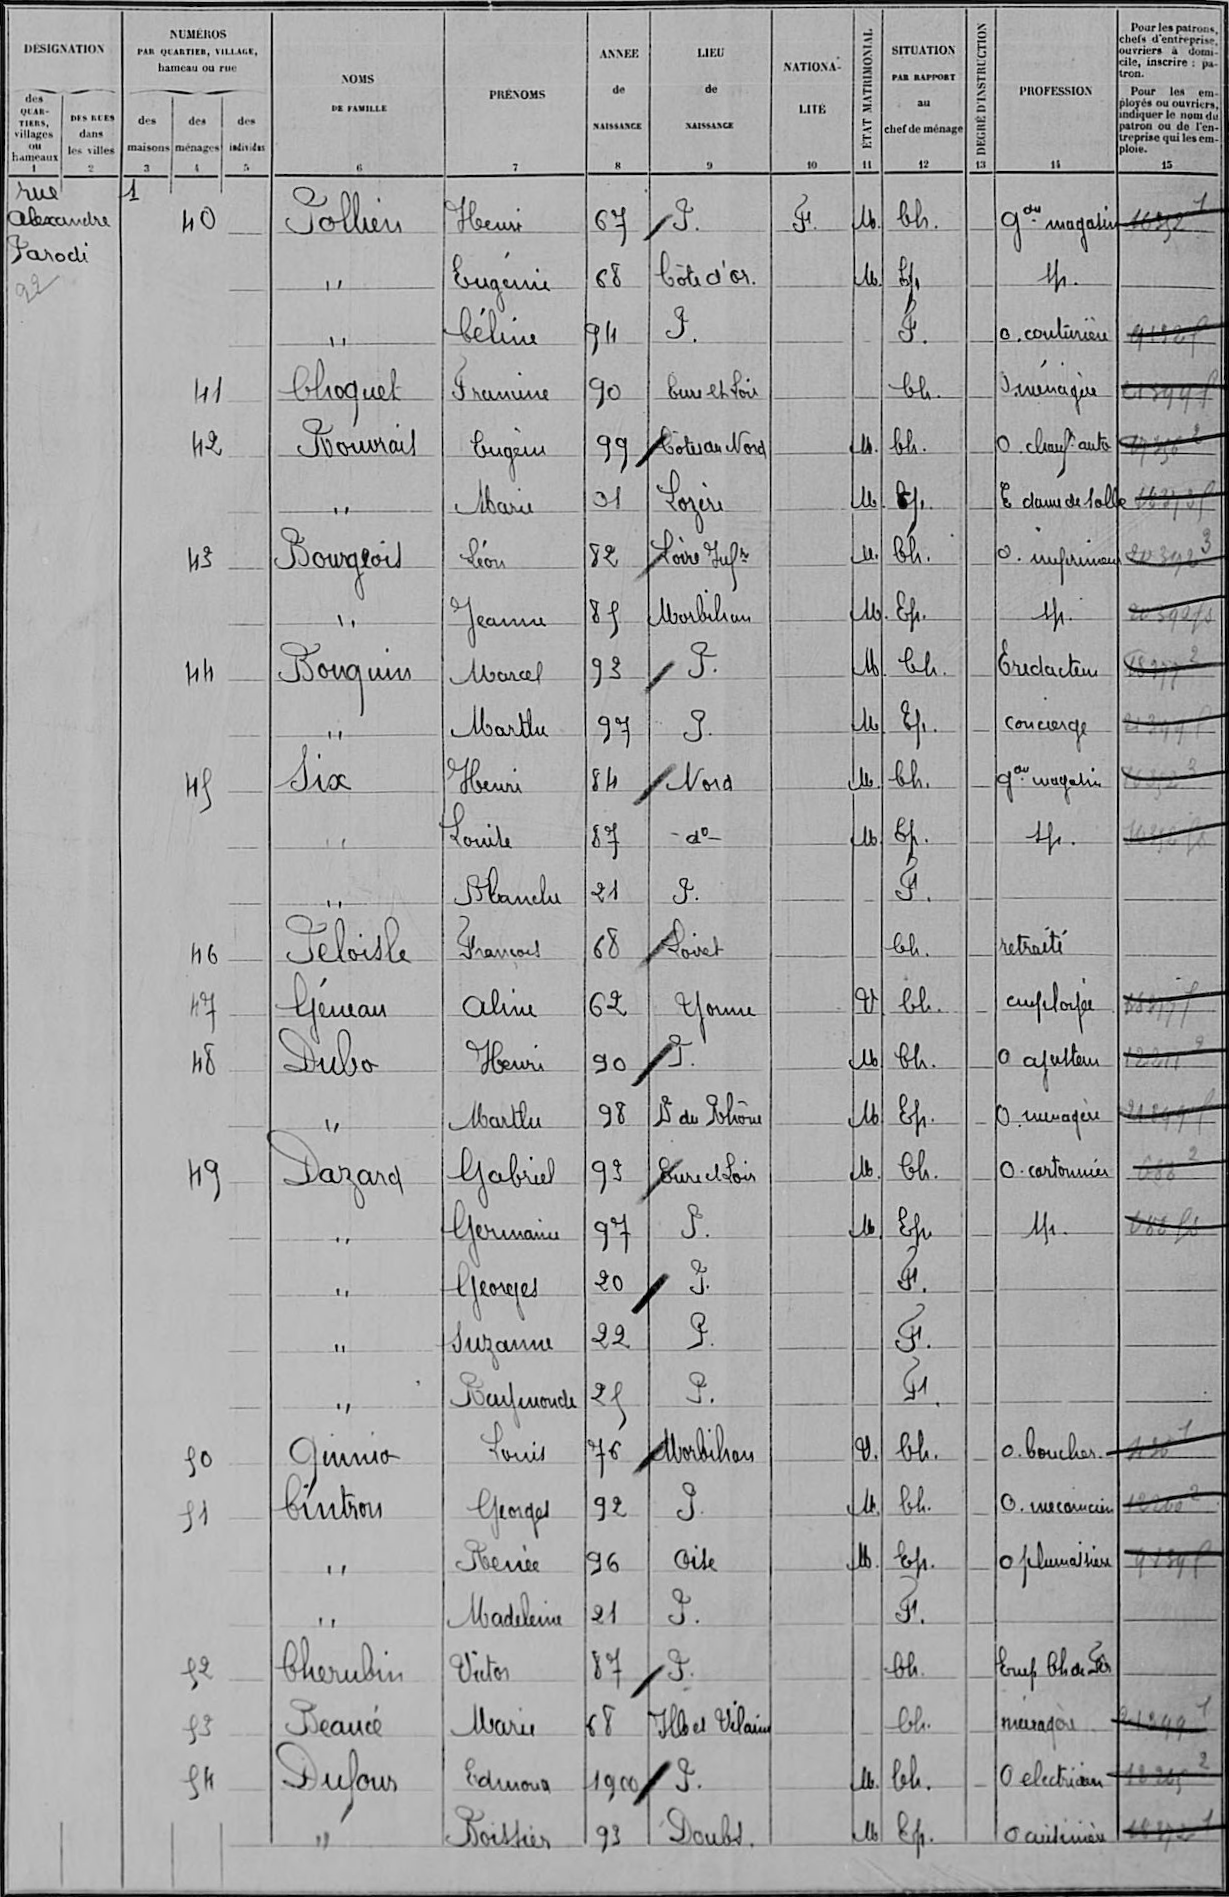Pollien/Henri/67/P /F /M /Ch /¤/Gon magasin/16352
"/Eugénie/68/Côte d'Or/¤/M /Ep/¤/sp /¤
¤/Céline/94/P /¤/¤/F /¤/o couturière/9132 f
Choquet/Francine/90/Eure et Loir/¤/¤/Ch /¤/o ménagère/21399 f
Rouvrais/Eugène/99/Côtes du Nord/¤/M /Ch /¤/O chauf auto/17356
¤/Marie/01/Lozère/¤/M /Ep /¤/E dame d salle/18373
Bourgeois/Léon/82/Loire Infre/¤/M/Ch /¤/o imprimeur/20392
¤/Jeanne/85/Morbihan/¤/M/Ep /¤/sp /20399 fs
Bouquin/Marcel/93/P /¤/M/Ch /¤/E redacteur/18377
¤/Marthe/97/P /¤/M/Ep /¤/concierge/21399 f
Six/Henri/84/Nord/¤/M /Ch /¤/gon magasin/16352
¤/Louise/87/d°/¤/M/Ep /¤/sp /16352
¤/Blanche/21/P /¤/¤/F /¤/¤/¤
Peloisle/François/68/Loiret/¤/¤/Ch /¤/retraité/¤
Géneau/Aline/62/Yonne/¤/V/Ch /¤/employée/18377
Dubo/Henri/90/P /¤/M /Ch /¤/o ajusteur/12277
¤/Marthe/98/B du Rhône/¤/M/Ep /¤/O menagere/21399 f
Dazard/Gabriel/93/Eure et Loir/¤/M /Ch /¤/O cartonnier/688
¤/Germaine/97/P /¤/M/Ep/¤/Sp /688 fs
"/Georges/20/P /¤/¤/F /¤/¤/¤
¤/Suzanne/2/P /¤/¤/F /¤/¤/¤
¤/Raymonde/25/P /¤/¤/F /¤/¤/¤
Ginnio/Louis/76/Morbihan/¤/V /Ch /¤/o boucher/436
Cintron/Georges/92/P /¤/M /Ch /¤/O mecanicien/12260
¤/Renée/96/Oise/¤/M /Ep /¤/o plumassière/9834 f
¤/Madeleine/21/P /¤/¤/F /¤/¤/¤
Cherubin/Victor/87/P /¤/¤/Ch /¤/Emp Ch de Fer/¤
Beaucé/Marie/68/Ille et Vilaine/¤/¤/Ch /¤/méangère/21399
Dufour/Edmond/1900/P /¤/M/Ch /¤/O elecricien/18205
¤/Boissier/93/Doubs/¤/M/Ep /¤/o cuisinière/18372
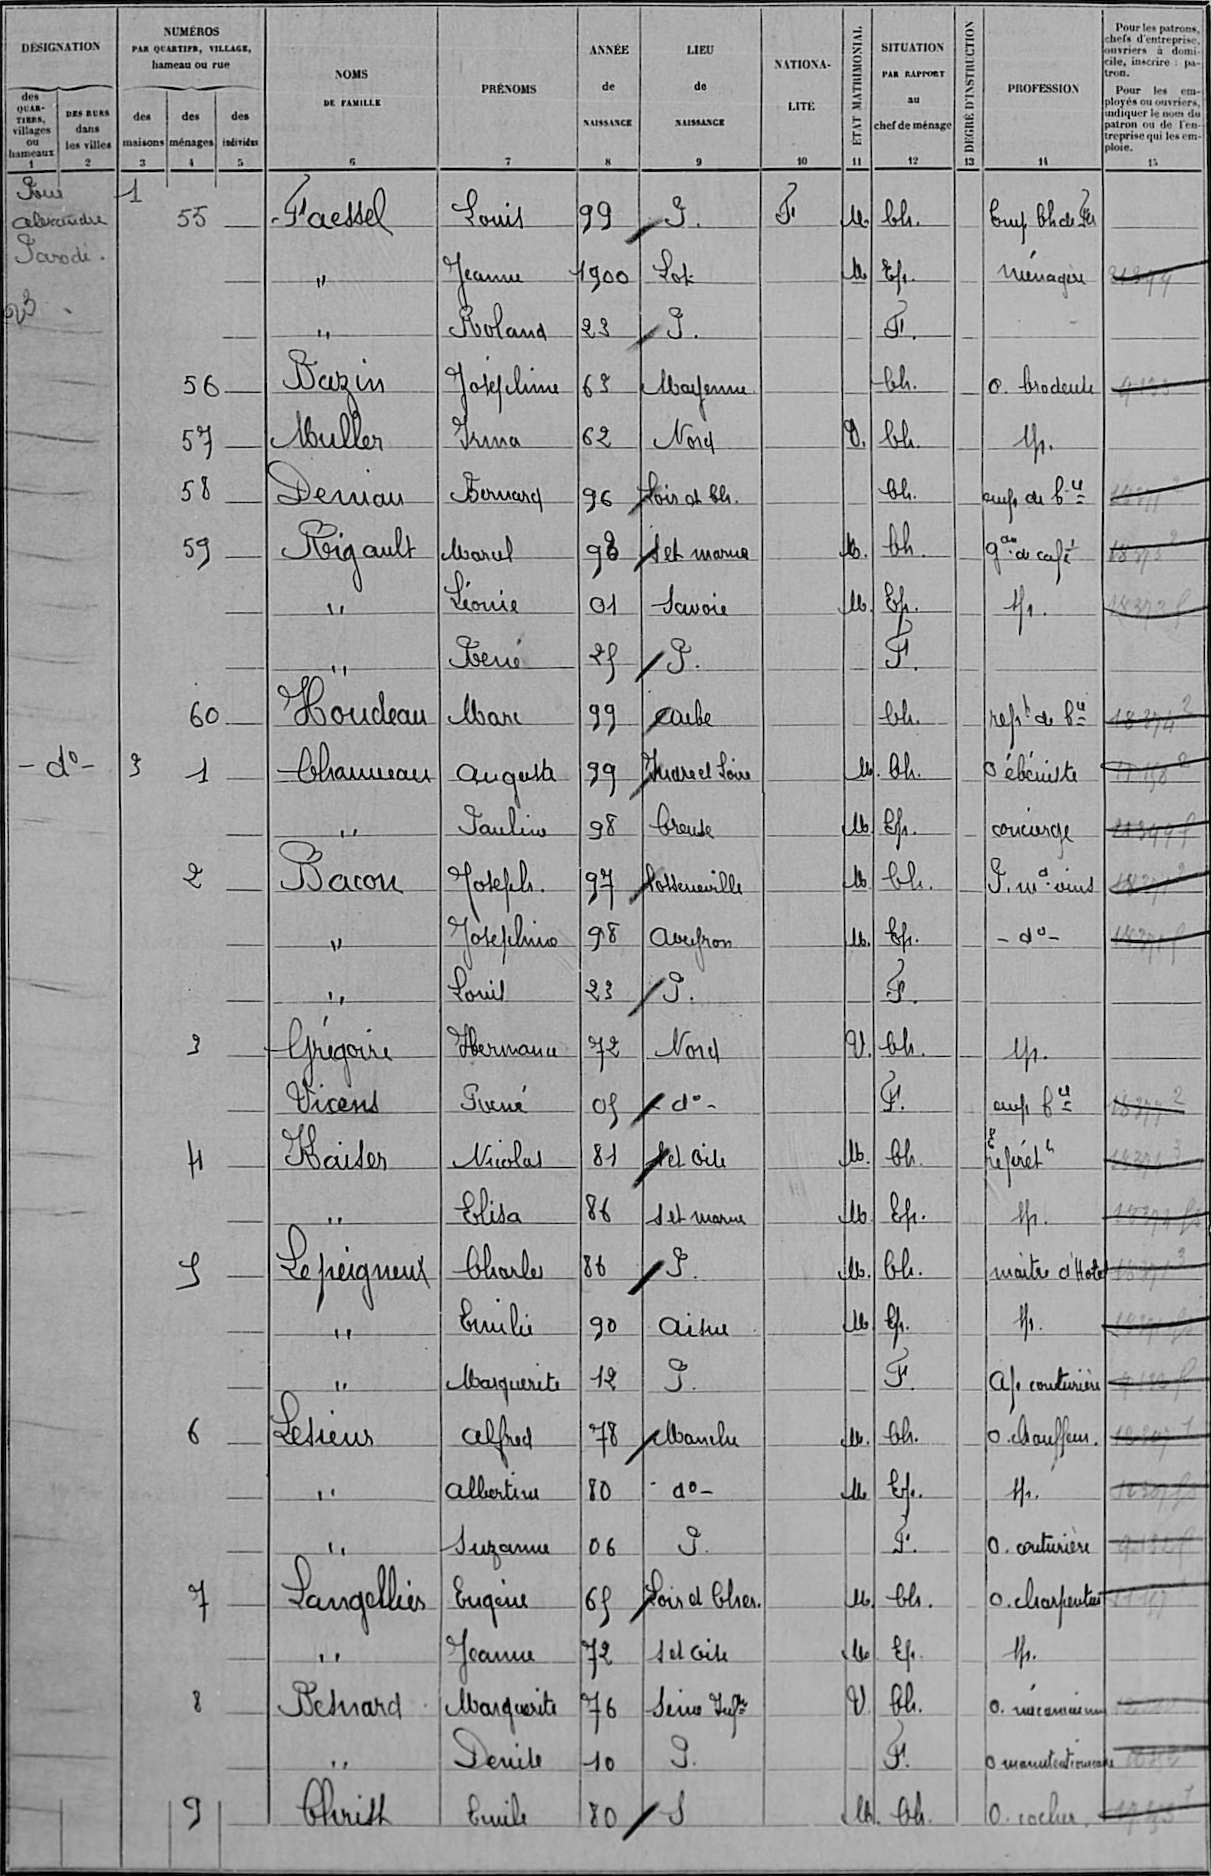Faessel/Louis/99/P /F/M /Ch /¤/Emp Ch de fer/¤
¤/Jeanne/1900/Lot/¤/M/Ep/¤/ménagère/21399
¤/Roland/23/P /¤/¤/F /¤/¤/¤
Bazin/Joséphine/63/Mayenne/¤/¤/Ch /¤/o brodeuse/9133
Muller/Irma/62/Nord/¤/D /Ch /¤/Sp /¤
Denian/Bernard/96/Loir et Ch /¤/¤/Ch /¤/emp de Cce/18877
Rigault/Marcel/98/S et marne/¤/M /Ch /¤/gon de café/18873
¤/Léonit/01/Savoie/¤/M/Ep /¤/Sp /18373
¤/René/25/P /¤/¤/F /¤/¤/¤
Houdeau/Marc/99/Aube/¤/¤/Ch /¤/repé de Cce/18374
Chauneau/Auguste/99/Indre et Loire/¤/M /Ch /¤/P ébéniste/17158
¤/Pauline/98/Creuse/¤/M/Ep /¤/concierge/21399 f
Bacon/Joseph/97/Posseneville/¤/M/Ch /¤/P md vins/18371
¤/Joséphine/98/Aveyron/¤/M /Ep /¤/d°/18371 f
¤/Louis/23/P /¤/¤/F /¤/¤/¤
Grégoire/Hermance/72/Nord/¤/V /Ch /¤/Sp /¤
Vicens/René/05/d°/¤/¤/F /¤/emp Cce/18377
Kaiser/Nicolas/81/S et Oise/¤/M /Ch /¤/E représt/18374
¤/Elisa/86/S et marne/¤/M/Ep /¤/Sp /18394
Lepeigneux/Charles/86/P /¤/M /Ch /¤/maitre d'Hotel/18371
¤/Emilie/90/Aisne/¤/M/Ep/¤/Sp /18371 fs
¤/Marguerite/12/P /¤/¤/F /¤/ap couturière/9132 f
Lesieur/Alfred/78/Manche/¤/M /Ch /¤/o chauffeur/12307
¤/Albertine/80/d°/¤/M/Ep /¤/Sp /12307 fs
¤/Suzanne/06/P /¤/¤/F /¤/o couturière/9132 f
Langellier/Eugène/65/Loir et Cher/¤/M /Ch /¤/o charpentier/11157
¤/Jeanne/72/S et Oise/¤/M/Ep /¤/Sp /¤
Besnard/Marguerite/76/Seine Infre/¤/V /Ch /¤/o mécanicienne/12260
¤/Denise/10/P /¤/¤/F /¤/o manutentionnaire/16352
Christ/Emile/80/S/¤/M/Ch /¤/O cocher/17353